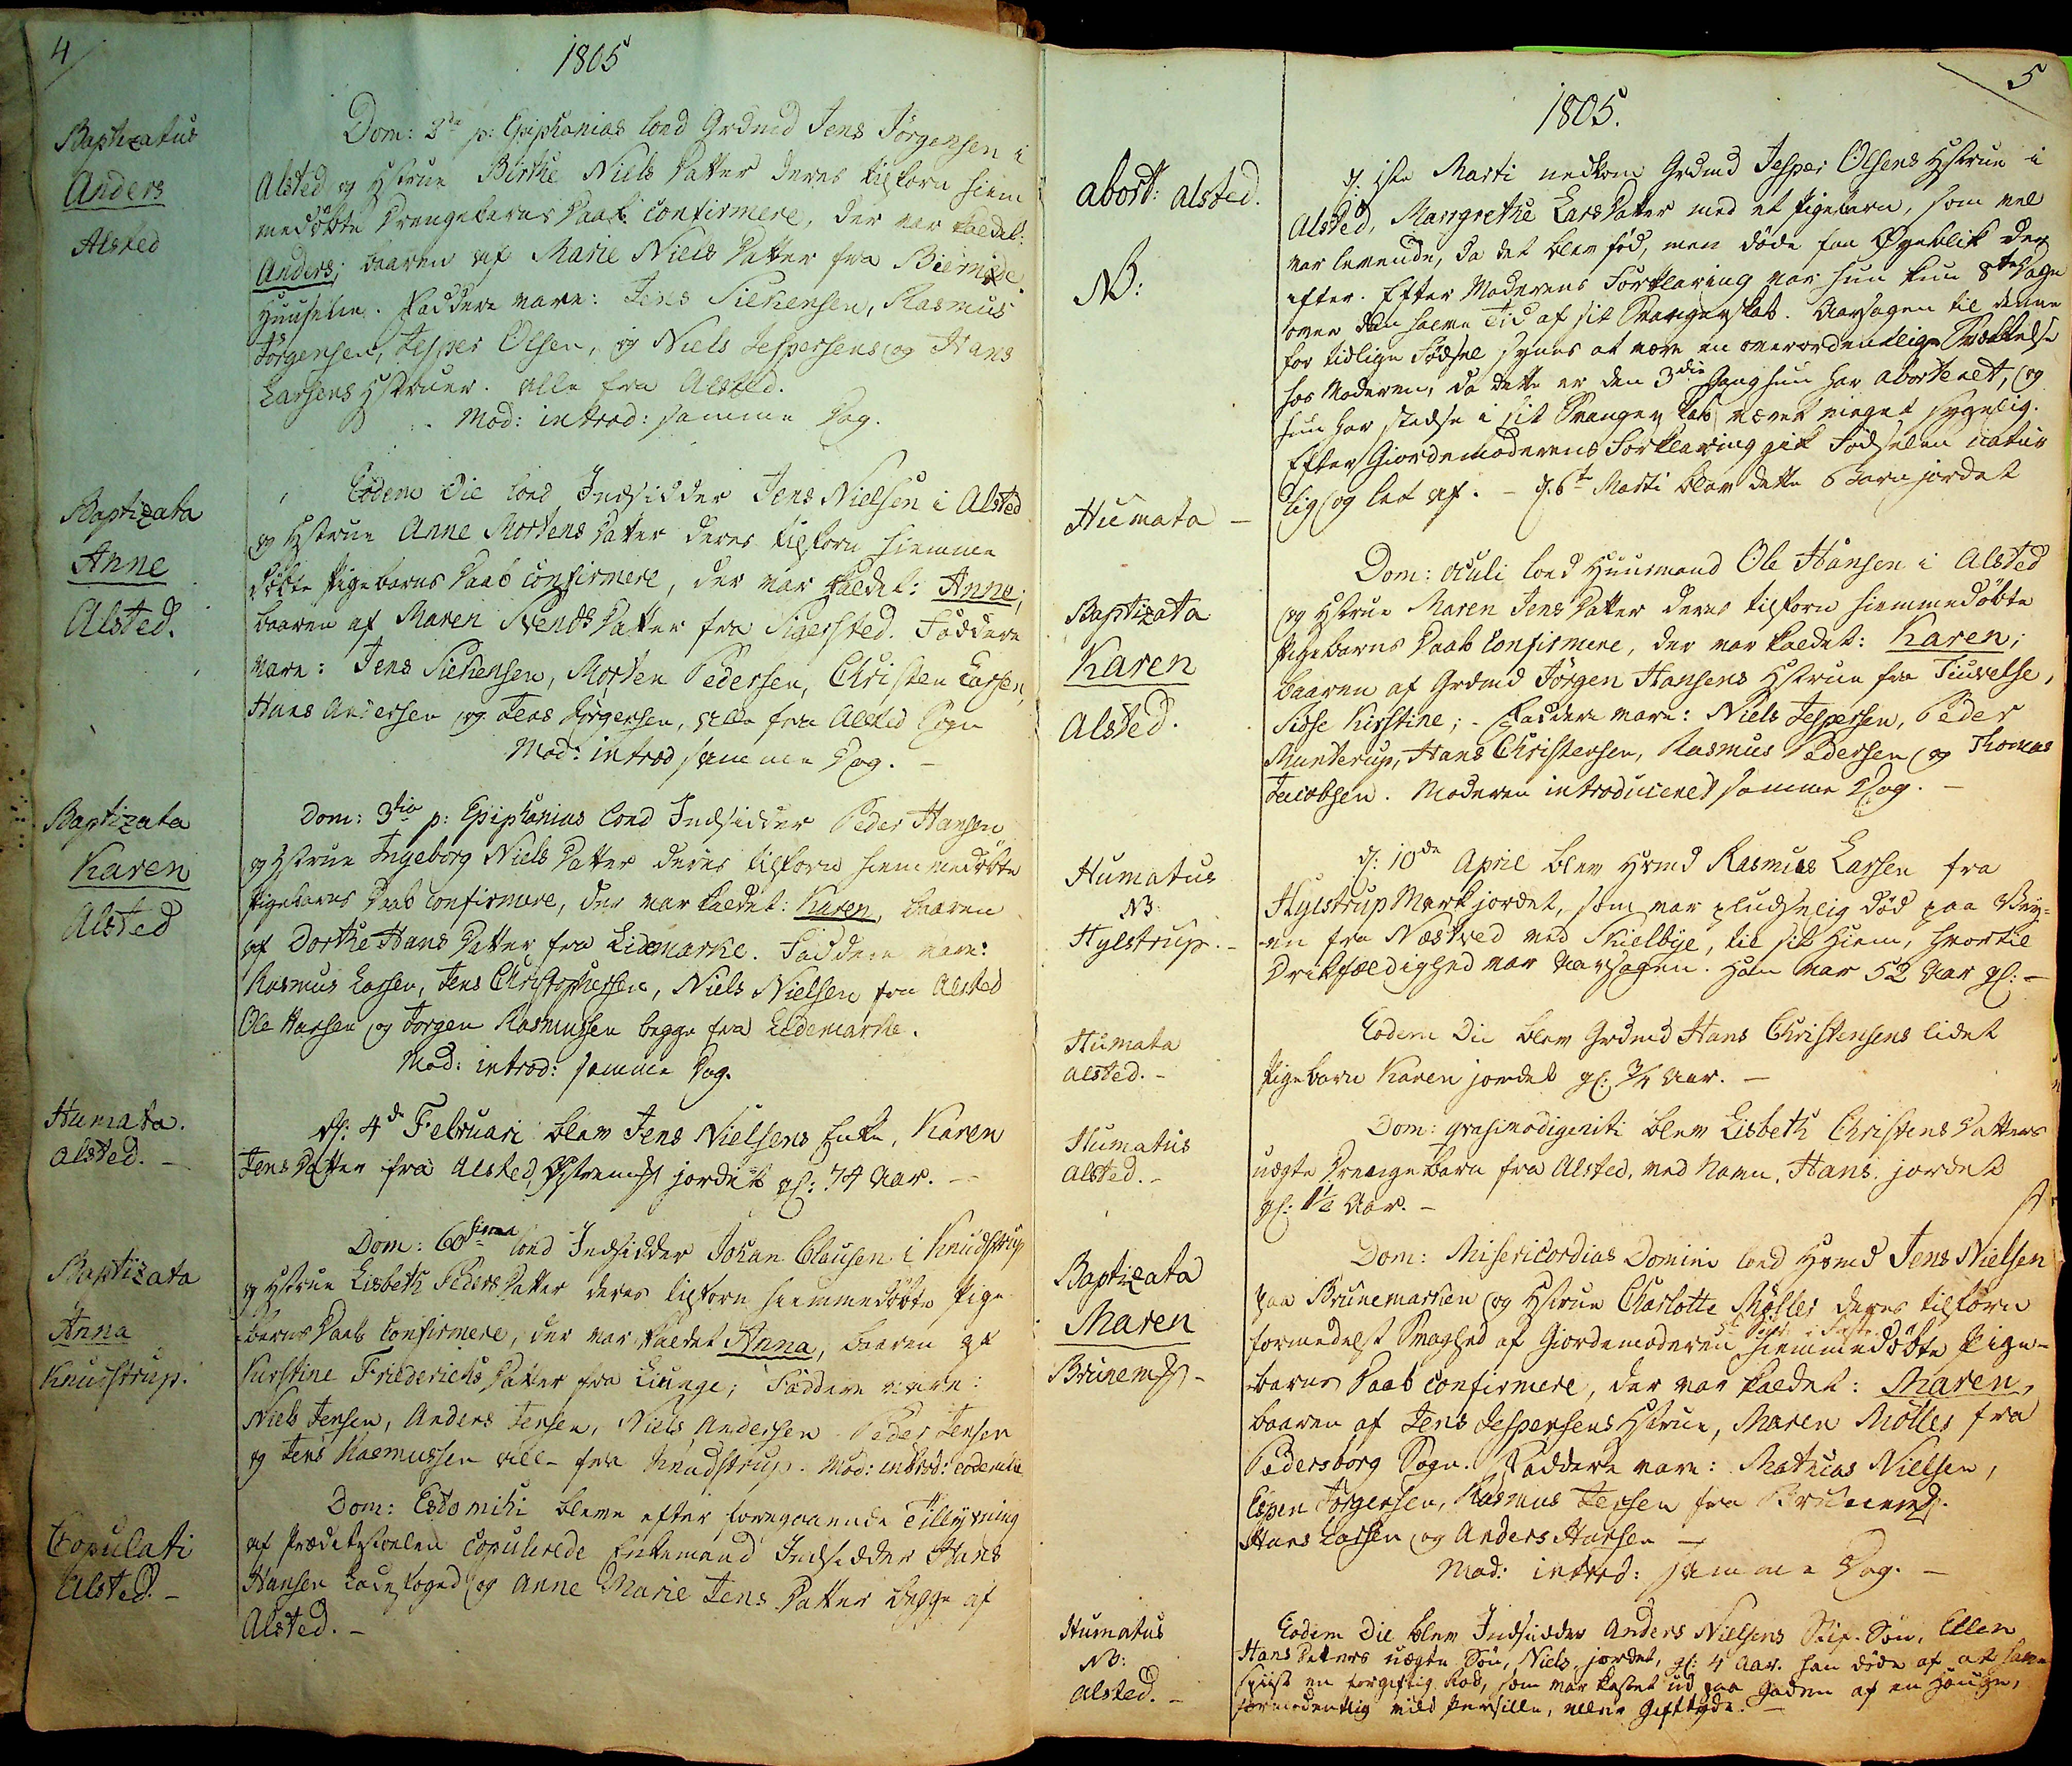4
Baphizatus
Anders
Alsted
Baptizata
Anne
Alsted
Baptizata
Karen
Alsted
Humata.
Alsted.
Baptizata
Anna
Knudstrup.
Copulati
Alsted.
1805
Dom: 2de p: Epiphanias loed Grdmd Jens Jørgensen i
Alsted og Hstue Birthe Niels Datter deres tilforn hiem¬
medøbte Drengebarns Daab confirmere, der var kaldet
Anders, baaren af Marie Niels Datter fra Biernede
Huusen. Faddere vare: Jens Silkensen, Rasmus
Jørgensen, Jesper Olsen, og Niels Jespersens og Hans
Larsens Hstruer. alle fra Alsted.
Mod: introd: samme Dag.
Eodem Die loed Indsidder Jens Nielsen i Alsted
og Hstrue Anne Mortens Datter deres tilforn hiemme
døbte Pigebarns Daab confirmere, der var kaldet: Anne;
baaren af Maren Svends Datter fra Sigersted. Faddere
vare: Jens Silkensen, Morten Pedersen, Christen Larsen
Hans Andersen og Jens Jørgensen, alle fra Alsted Sogn
Mod: introd samme Dag.
Dom: 3tia p. Epiphanias loed Indsidder Peder Hansen
og Hstrue Ingeborg Niels Datter deres tilforn hiemmedøbte
Pigebarns Daab confirmere, der var kaldet: Karen, baaren
af Dorthe Hans Datter fra Lidemarke. Faddere vare
Rasmus Larsen, Jens Christophersen, Niels Nielsen fra Alsted
Ole Hansen og Jørgen Rasmussen begge fra Lidemarke.
Mod: introd: samme Dag.
d: 4de Februari blev Jens Nielsens Enke, Karen
Jens Datter fra Alsted Østrem## jordet gl: 74 Aar.
og Hstrue Lisbeth Peders Datter deres tilforn hiemmedøbte Pige
Dom: 60sima  loed Indsidder Johan Clausen i Knudstrup
barns Daab confirmeret, der var kaldet Anna; baaren af
Kirstine Friderichs Datter fra Liunge; Faddere vare:
Niels Jensen, Anders Jensen, Niels Andersen, Peder Jensen
og Jens Rasmussen alle fra Knudstrup. Mod: introd: Eodem##
Dom: Esto mihi bleve efter foregaaende Tillysning
af Prædikestolen copulerede Enkemand Indsidder Hans
Hansen Ladefoged og Anne Marie Jens Datter begge af
Alsted.
abort: Alsted
NB
Humata
Baptizata
Karen
Alsted
Humatus
NB
Hylstrup
Humata
Alsted.
Humatus.
Alsted.
Baptizata
Maren
Brunem##.
Humatus
NB.
Alested.
5
1805.
d. 1ste Marti nedkom Grdmd Jesper Olsens Hustrue i
Alsted, Margrethe Lars Datter med et Pigebarn, som vel
var levende, da det blev fød, men døde saa Øgeblik der
efter. Efter Moderens Forklaring var hun kun 8te Dage
over den halve Tid af sit Svangerskab. Aarsagen til denne
for tidlige Fødsel synes at være en overordendlige Svækkelse
hos Moderen, da dette er den 3die gang hun har aborteret, og
hun har stedse i sit Svangenskab været meget sygelig.
Efter Giordemoderens Forklaring gik Fødselen natur
lig og let af . - d. 6te Marti blev dette Barn jordet
Dom: Oculi loed Huusmand Ole Hansen i Alsted
og Hstrue Maren Jens Datter deres tilforn hiemmedøbte
Pigebarns Daab confirmere, der var kaldet: Karen;
baaren af Grdmd Jørgen Hansens Hstrue fra Tiuvelse,
Sidse Kirstine; Faddere vare: Niels Jespersen, Peder
Munterup; - Hans Christensen, Rasmus Pedersen og Thomas
Jacobsen. Moderen introduceret samme Dag.
d: 10de April blev Hsmd Rasmus Larsen fra
Hylstrup Mark jordet, som var pludselig død paa Vey¬
en fra Næstved ved Skielbye, til sit Hiem, hvortil
Drikfældighed var Aaarsagen. Han var 52 Aar gl:
Eodem Die blev Grdmd Hans Christensens lidet
Pigebarn Karen Jordet gl: ¾ Aar.
Dom: Qvasimodogeniti blev Lisbeth Christens Datters
uægte Drengebarn fra Alsted, ved navn Hans. jordet
gl: 1½ Aar.
Dom: Misericordias Domini loed Hsmd Jens Nielsen
paa Brunemarken og Hstrue Charlotte Møller deres tilforn
5te Sønd: i Faste
formedelst Svaghed af Giordemoderen hiemmedøbte Pige¬
barns Daab confirmere, der var kaldet: Maren
baaren af Jens Jespersens Hstrue, Maren Møller fra
Pederborg Sogn. Faddere vare: Mathias Nielsen
Espen Jørgensen, Rasmus Jensen fra Brune##
Hans Larsen og Anders Hansen
Mod: introd: samme Dag.
Eodem Die blev Indsidder Anders Nielsens Stif. Søn, Ellen
Hans Datters uægte Søn, Niels jordet gl: 4 Aar. han døde af at have
spiist en forgiftigRod, som var kastet ud paa Gaden af en Hauge,
formedentlig vild Persille, eller Gifttyde.
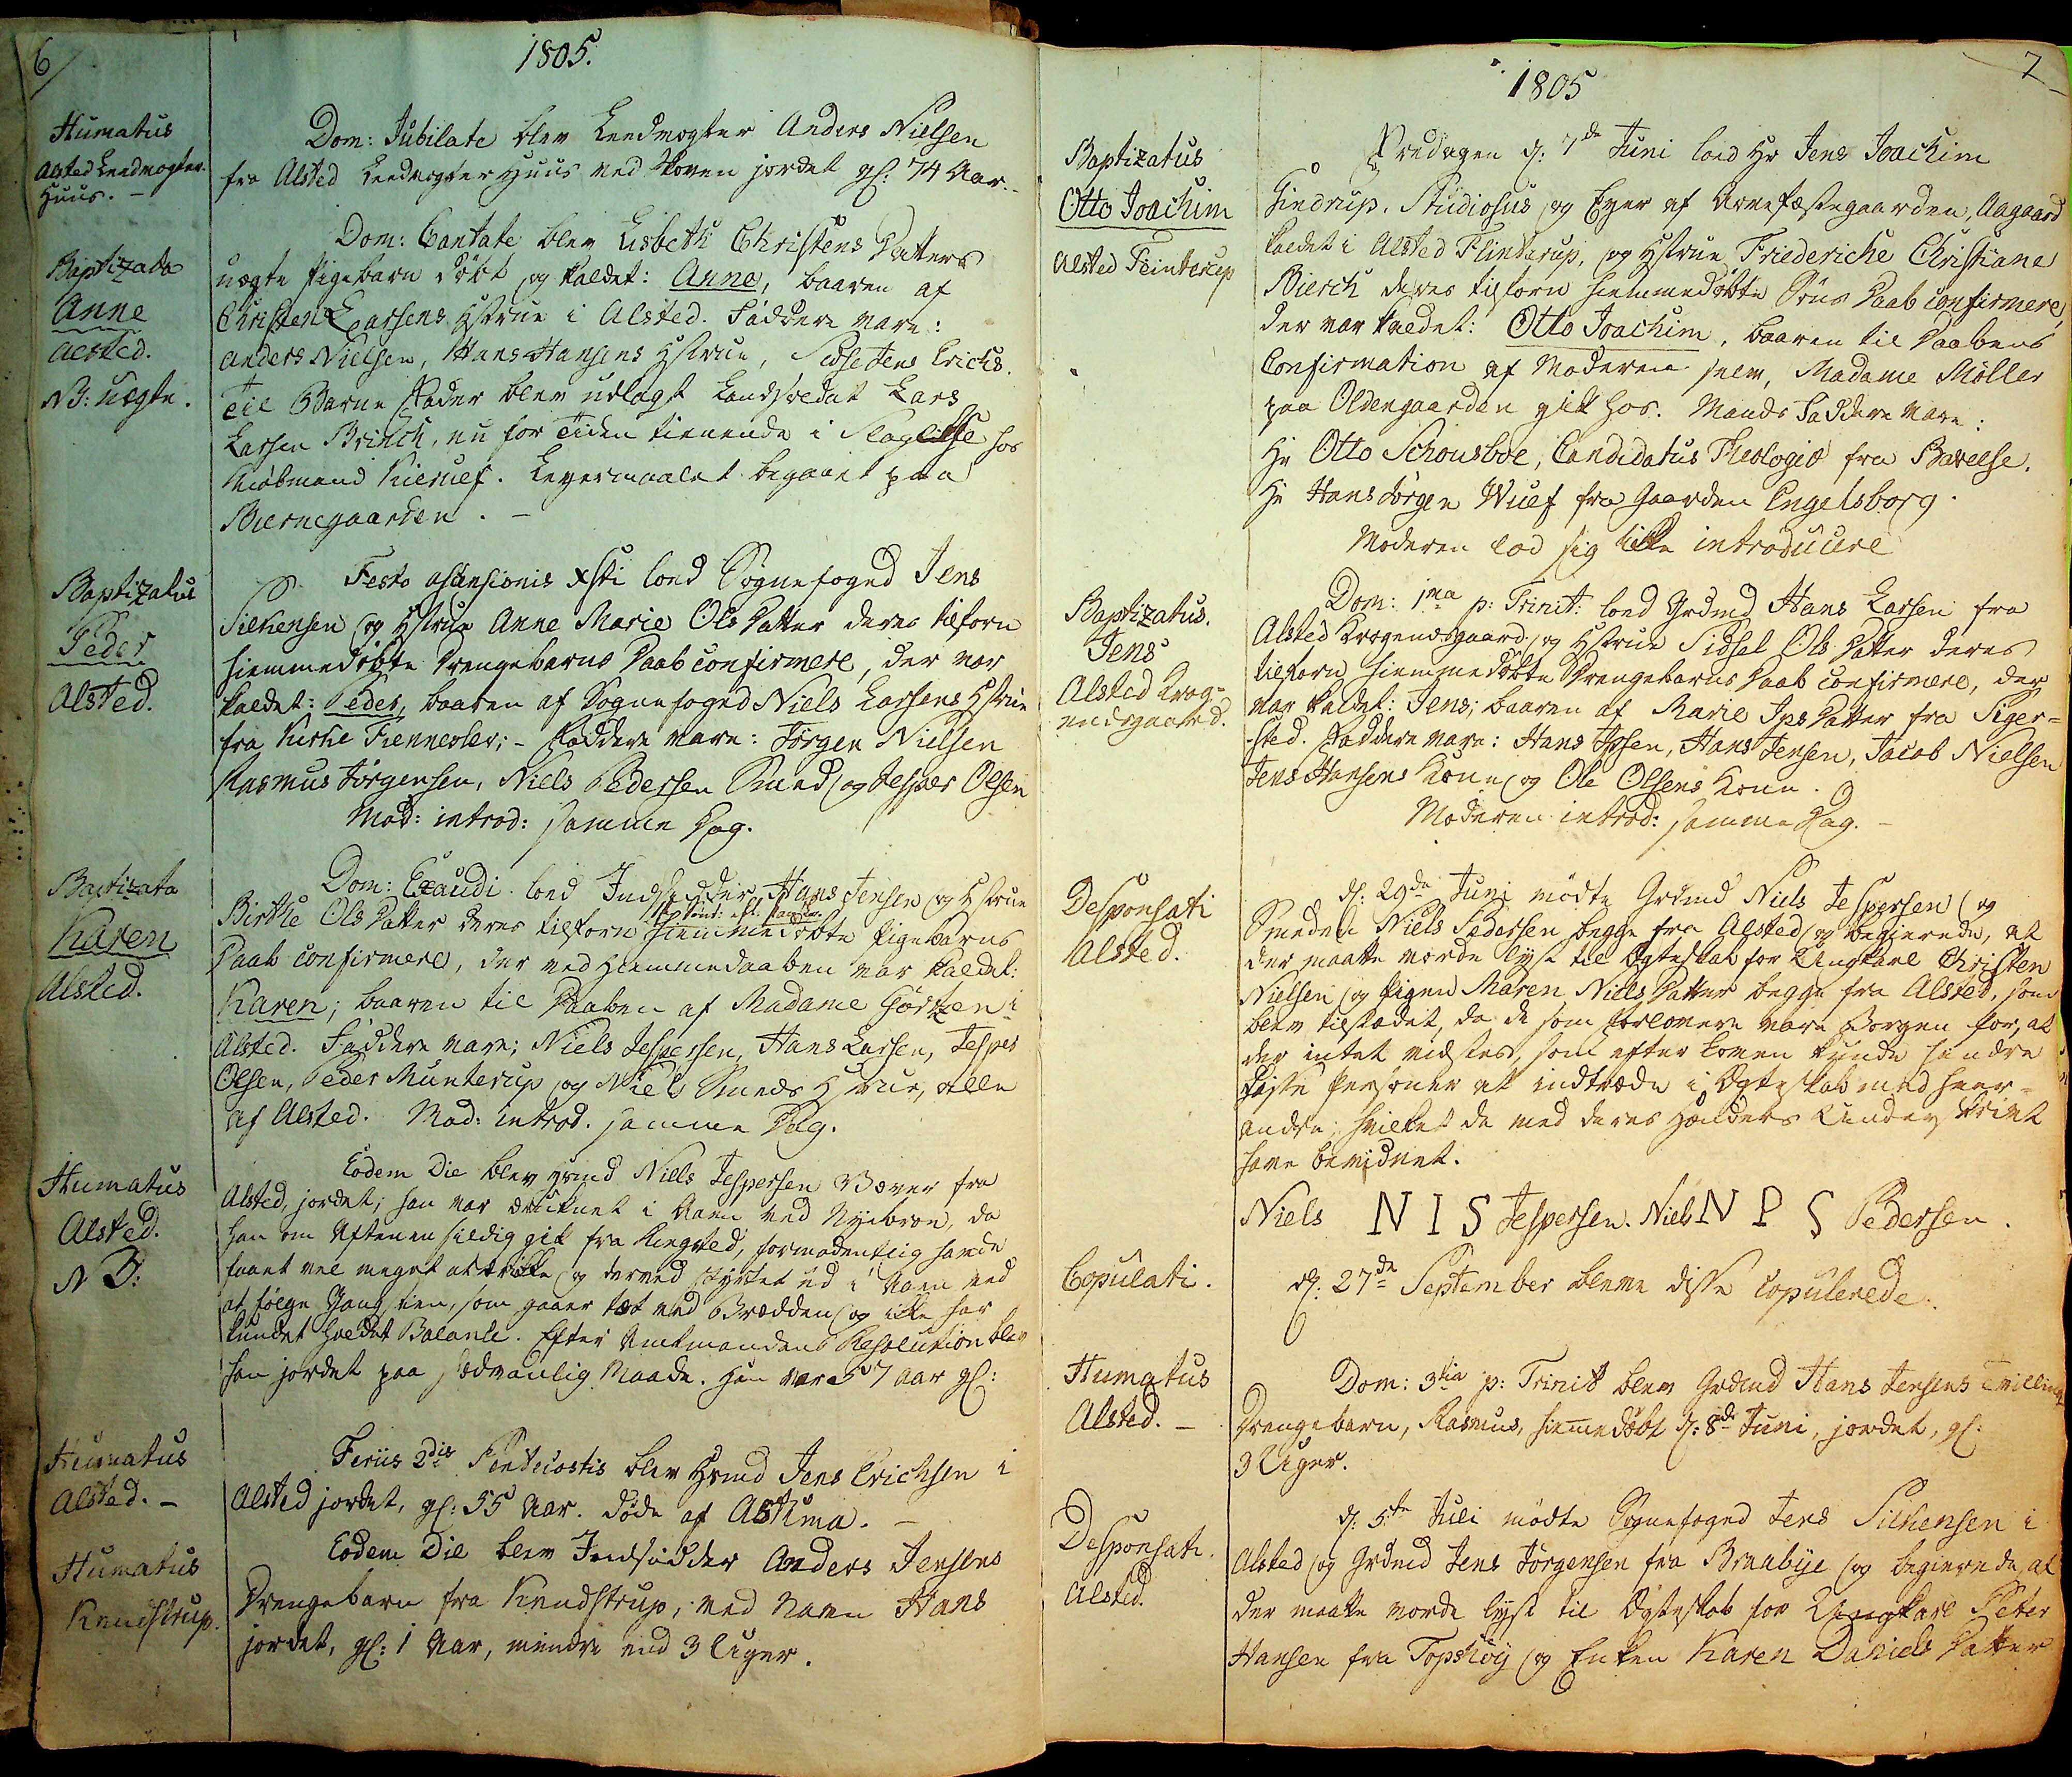6/
Humatus
Alsted Leedvogter
Huus.
Baptizata
Anne
Alsted
NB: uægte.
Baptizatus
Peder
Alsted.
Baptizata
Karen
Alsted.
Humatus
Alsted
NB:
Humatus
Alsted.
Humatus
Knudstrup
1805.
Dom: Jubilate blev Leedvogter Anders Nielsen
fra Alsted Leedvogter Huus. ved Skoven jordet gl: 74 Aar.
Dom: Cantate blev Lisbeth Christens Datters
uægte Pigebarn døbt og kaldet Anne, baaren af
Christen Larsens Hstrue i Alsted. Faddere vare:
Anders Nielssen, Hans Hansens Hstrue, Sidse Jens Erichs.
Til Barne Fader blev udlagt Landsoldat Lars
Larsen Briech, nu for Tiden tienende i Slagelse hos
Kiøbmand Kierulf. Legermalet begaaet paa
Bieregaarden.
Festo Ascensionis Xsti loed Sognefoged Jens
Silkensen og Hstrue Anne Marie Ols Datter deres tilforn
hiemmedøbte Drengebarns Daab confirmere, der var
kaldet: Peder, baaren af Sognefoged Niels Larsens Hstrue
fra Kirke Fienneslev; - Faddere vare: Jørgen Nielsen
Rasmus Jørgensen, Niels Pedersen Smed, og Jesper Olsen
Mod: introd: samme Dag.
Dom: Exaudi loed Indsidder Hans Jensen og Hstrue
1ste Sønf: eft. Paaske
Birthe Ols Datter deres tilforn hiemmedøbte Pigebarns
Daab confirmere, der ved Hiemmedaaben var kaldet:
Karen; baaren til Daaben af Madame Gørtzen i
Alsled. Faddere vare: Niels Jespersen, Hans Larsen, Jeppe
Olsen, Peder Munterup og Niels Smeds Hstrue, alle
af Asted. Mod: introd. samme Dag.
Eodem Die blev Hsmd Niels Jespersen Væver fra
Alsted jordet; han var druknet i Aaen ved Nyebroe, da
han om Aftenen sldig gik fra Ringsted, formodentlig havde
faaet vel meget at drikke og derved styrtet ud i Aaen ved
at følge Gangstiuen, som gaaer tæt ved Brædder og ikke har
kundet holdet Balance. Efter Amtmandens Refsolution blev
han jordet paa sædvanlig Maade. Han var 57 Aar gl:
Feriis 2dis Pentecostis blev Hsmd Jens Erichsen i
Alsted jordet, gl: 55 Aar. døde af Astma.
Eodem Die blev Indsidder Anders Jensens
Drengebarn fra Knudstrup, ved Navn Hans
jordet, gl: 1 Aar, mindre end 3 Uger.
Baptizatus
Otto Joachim
Alsted Flinterup
Baptizatus.
Jens
Alsted Krog¬
endegaard
Desponsati
Alsted.
Copulati.
Humatus
Alsted
Desponsati.
Alsted.
7
1805
Fredagen d: 7de Juni loed Hr Jens Joachim
Giedrup Studiosus, og Eyer af Arvefæstegaarden, Aagaard
kaldet i Alsted Flinterup, og Hstrue Frideriche Christiane
Bierch deres tilforn hiemmedøbte Søns Daab confirmere
der var kaldet: Otto Joachum, baaren til Daabens
Confirmation af Moderen selv, Madame Møller
paa Oldengaarden gik hos. Mands Faddere vare:
Hr Otto Schousboe, Candidatus Theologie fra Bavelse,
Hr Hans Jørgen Wulf fra Gaarden Engelsborg
Moderen lod sig ikke introducere
Dom: 1ma p: Trinit: loed Grdmd Hans Larsen fra
Alsted Krogendegaard, og Hstrue Sidsel Ols Datter deres
tilforn hiemmedøbte Drengebarns Daab confirmere, der
var kaldet: Jens; baaren af Marie Ips Datter fra Siger¬
sted. Faddere vare: Hans Ipsen, Hans Jensen, Jacob Nielsen
Jens Hansens Kone og Ole Olsens Kone
Moderen introd: samme Dag.
d: 29de Juni mødte Grdmd Niels Jespersen og
Smeden Niels Pedersen begge fra Alsted og begierede, at
der maatte vorde lyst til Ægteskab for Ungkarl Christen
Nielsen og Pigen Maren Niels Datter begge fra Alsted, som
blev tilstædet, da de som Forlovere vare Borgen for, at
der intet vidstes, som efter Loven kunde hindre
disse Personer at intræde i Ægteskab med hver¬
andre, hvilket de med deres Hænders Underskrivt
have bevidnet.
Niels N I S Jespersen. Niels N P S Pedersen.
d. 27de September bleve disse copulerede.
Dom: 3tia p: Trinit blev Grdmd Hans Jensens Tvilling
Drengebarn, Rasmus, hiemmedøbt d: 8de Juni, jordet gl:
3 Uger.
d: 5te Juli mødte Sognefoged Jens Silkensen i
Alsted og Grdmd Jens Jørgensen fra Braabye og begierede at
der maatte vorde lyst til Ægteskab for Ungkarl Peter
Hansen fra Topshøy og Enken Karen Daniels Datter